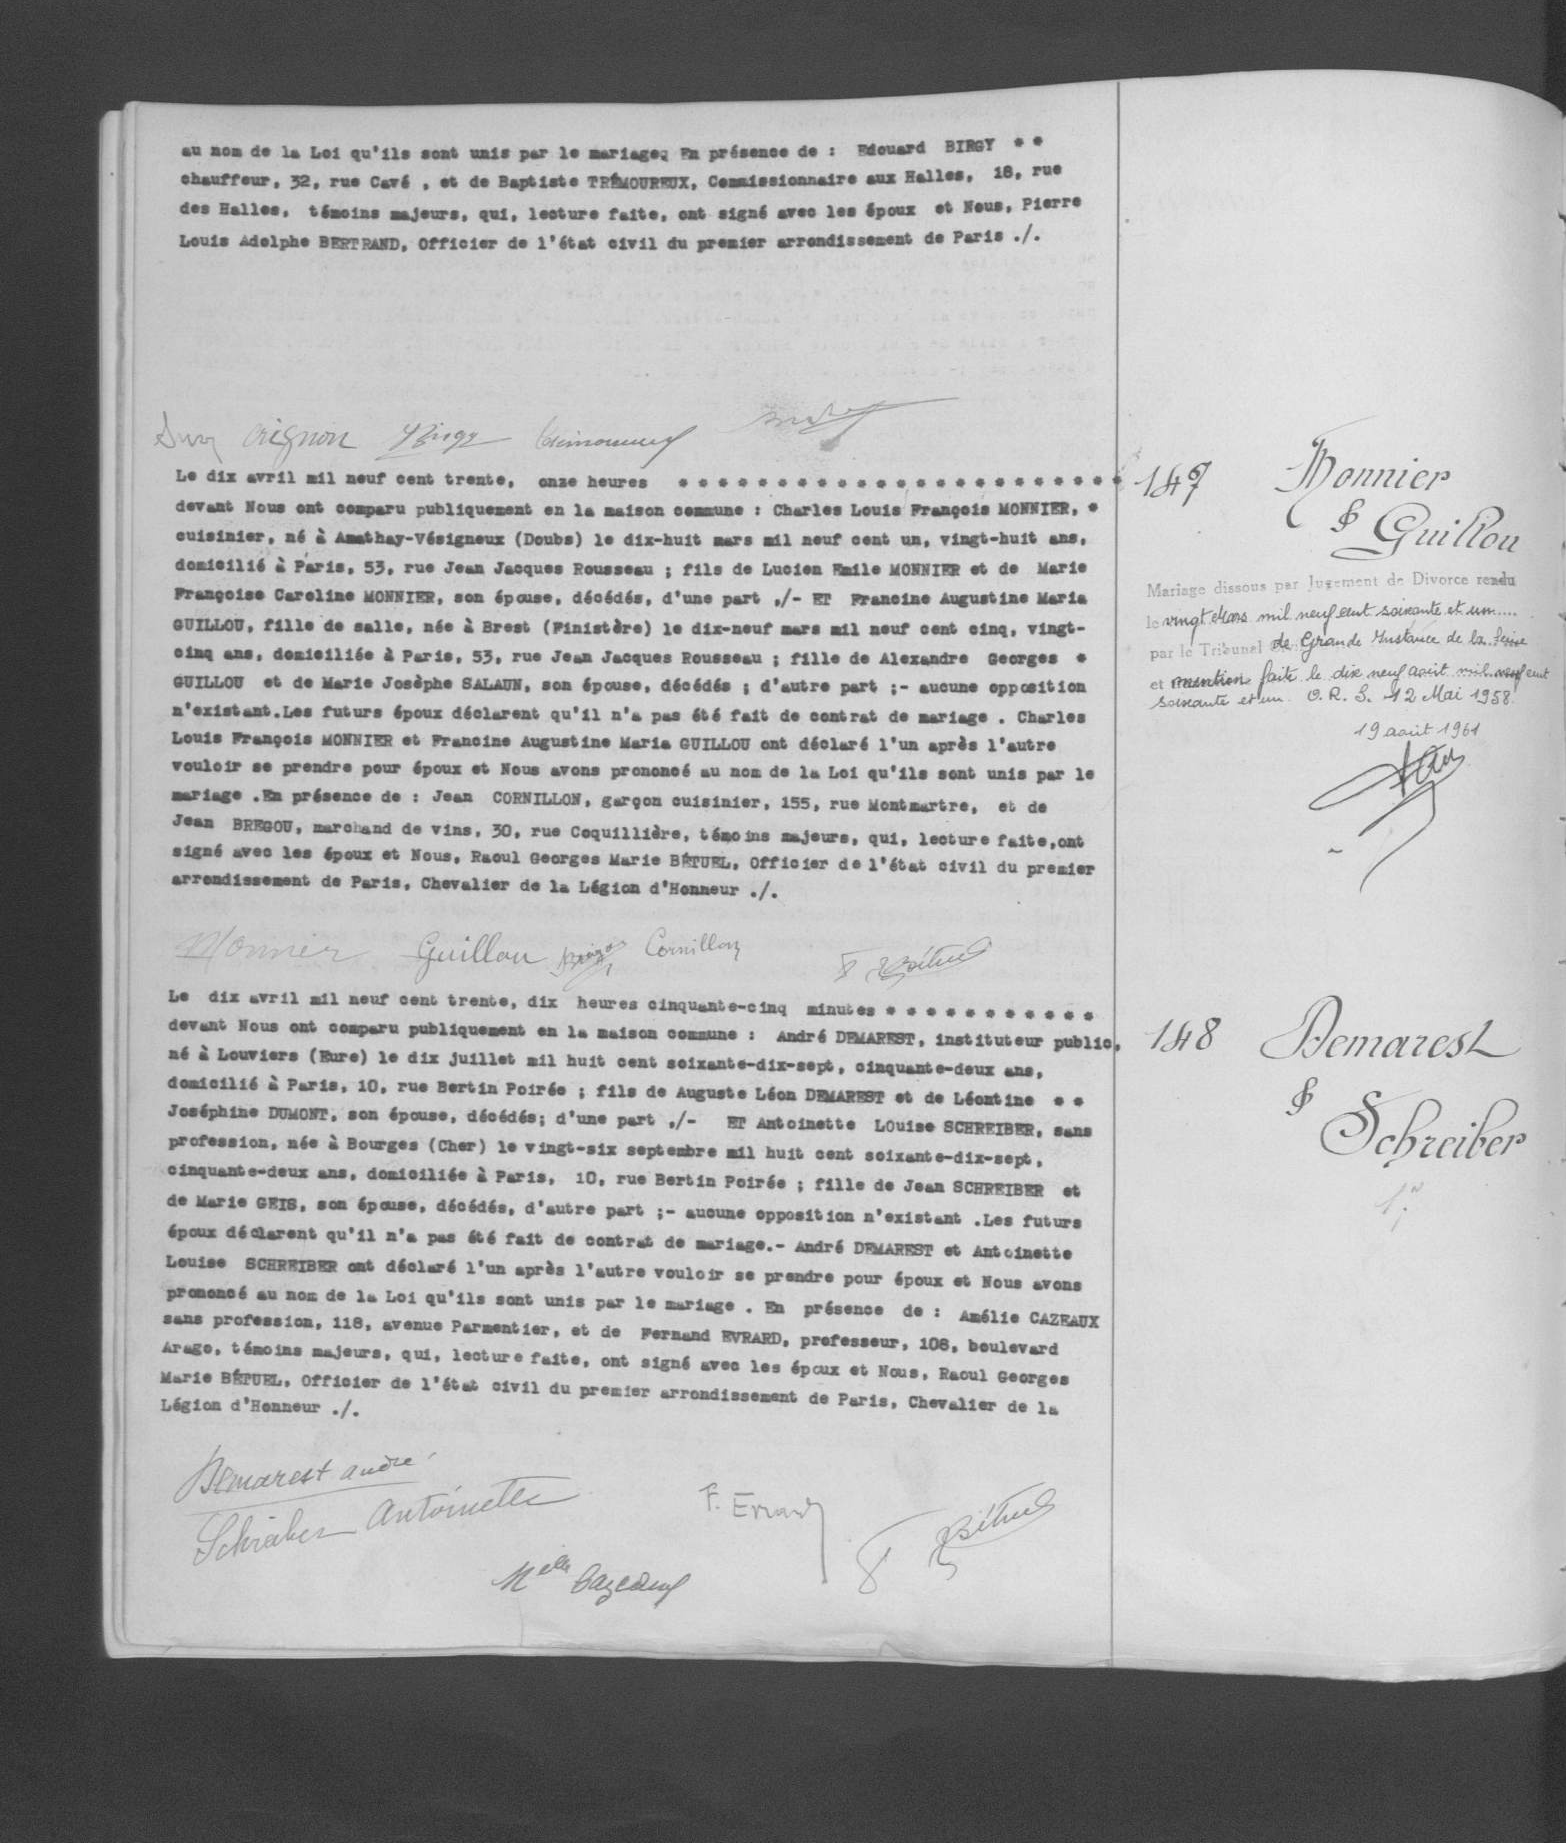au nom de la Loi qu'ils sont unis par le mariage. En présence de: Edouard BIRGY * *
chauffeur, 32, rue Cavé, et de Baptiste TREMOUREUX, Comissionnaire aux Halles, 18, rue
des Halles, témoins majeurs, qui, lecture faite, ont signé avec les époux et Nous, Pierre
Louis Adolphe BERTRAND, Officier de l'état civil du premier arrondissement de Paris ./.
Le dix avril mil neuf cent trente, onze heures ****
devant Nous ont comparu publiquement en la maison commune: Charles Louis François MONNIER, *
cuisinier, né à Amathay-Vésigneux (Doubs) le dix-huit mars mil neuf cent un, vingt-huit ans,
domicilié à Paris, 53, rue Jean Jacques Rousseau; fils de Lucien Emile MONNIER et de Marie
Françoise Caroline MONNIER, son épouse, décédés, d'une part ,/-ET Francine Augustine Maria
GUILLOU, fille de salle, née à Brest (Finistère) le dix-neuf mars mil neuf cent cinq, vingt-
cinq ans, domiciliée à Paris, 53, rue Jean Jacques Rousseau; fille de Alexandre Georges *
GUILLOU et de Marie Josèphe SALAUN, son épouse, décédés; d'autre part ;-aucune opposition
n'existant. Les futurs époux déclarent qu'il n'a pas été fait de contrat de mariage. Charles
Louis François MONNIER et Francine Augustine Maria GUILLOU ont déclaré l'un après l'autre
vouloir se prendre pour époux et Nous avons prononcé au nom de la Loi qu'il sont unis par le
mariage. En présence de: Jean CORNILLON, garçon cuisinier, 155, rue Montmartre, et de
Jean BREGOU, marchand de vins, 30, rue Coquillière, témoins majeurs, qui, lecture faite, ont
signé avec les époux et Nous, Raoul Georges Marie BETUEL, Officier de l'état civil du premier
arrondissement de Paris, Chevalier de la Légion d'Honneur ./.
Le dix avril mil neuf cent trente, dix heures cinquante-cinq minutes ****
devant Nous ont comparu publiquement en la maison commune: André DEMAREST, instituteur public,
né à Louviers (Eure) le dix juillet mil huit cent soixante-dix-sept, cinquante-deux ans,
domicilié à Paris, 10, rue Bertin Poirée; fils de Auguste Léon DEMAREST et de Léontine * *
Joséphine DUMONT, son épouse, décédéx; d'une part ,/-ET Antoinette Louise SCHREIBER, sans
profession, née à Bourges (Cher) le vingt-six septembre mil huit cent soixante-dix-sept,
cinquante-deux ans, domiciliée à Paris, 10, rue Bertin Poiée; fille de Jean SCHREIBER et
de Marie GEIS, son épouse, décédés, d'autre part ;-aucune opposition n'existant. Les futurs
époux déclarent qu'il n'pas été fait de contrat de mariage .-André DEMAREST et Antoinette
Louise SCHREIBER ont déclaré l'un après l'autre vouloir se prendre pour époux et Nous avons
prononcé au nom de la Loi qu'ils sont unis par le mariage. En présence de: Amélie CAZEAUX
sans profession, 118, avenue Parmentier, et de Fernand EVRARD, professeur, 108, boulevard
Arage, témoins majeurs, qui, lecture faite, ont signé avec les époux et Nous, Raoul Georges
Marie BETUEL, Officier de l'état civil du premier arrondissement de Paris, Chevalier de la
Légion d'Honneur ./.
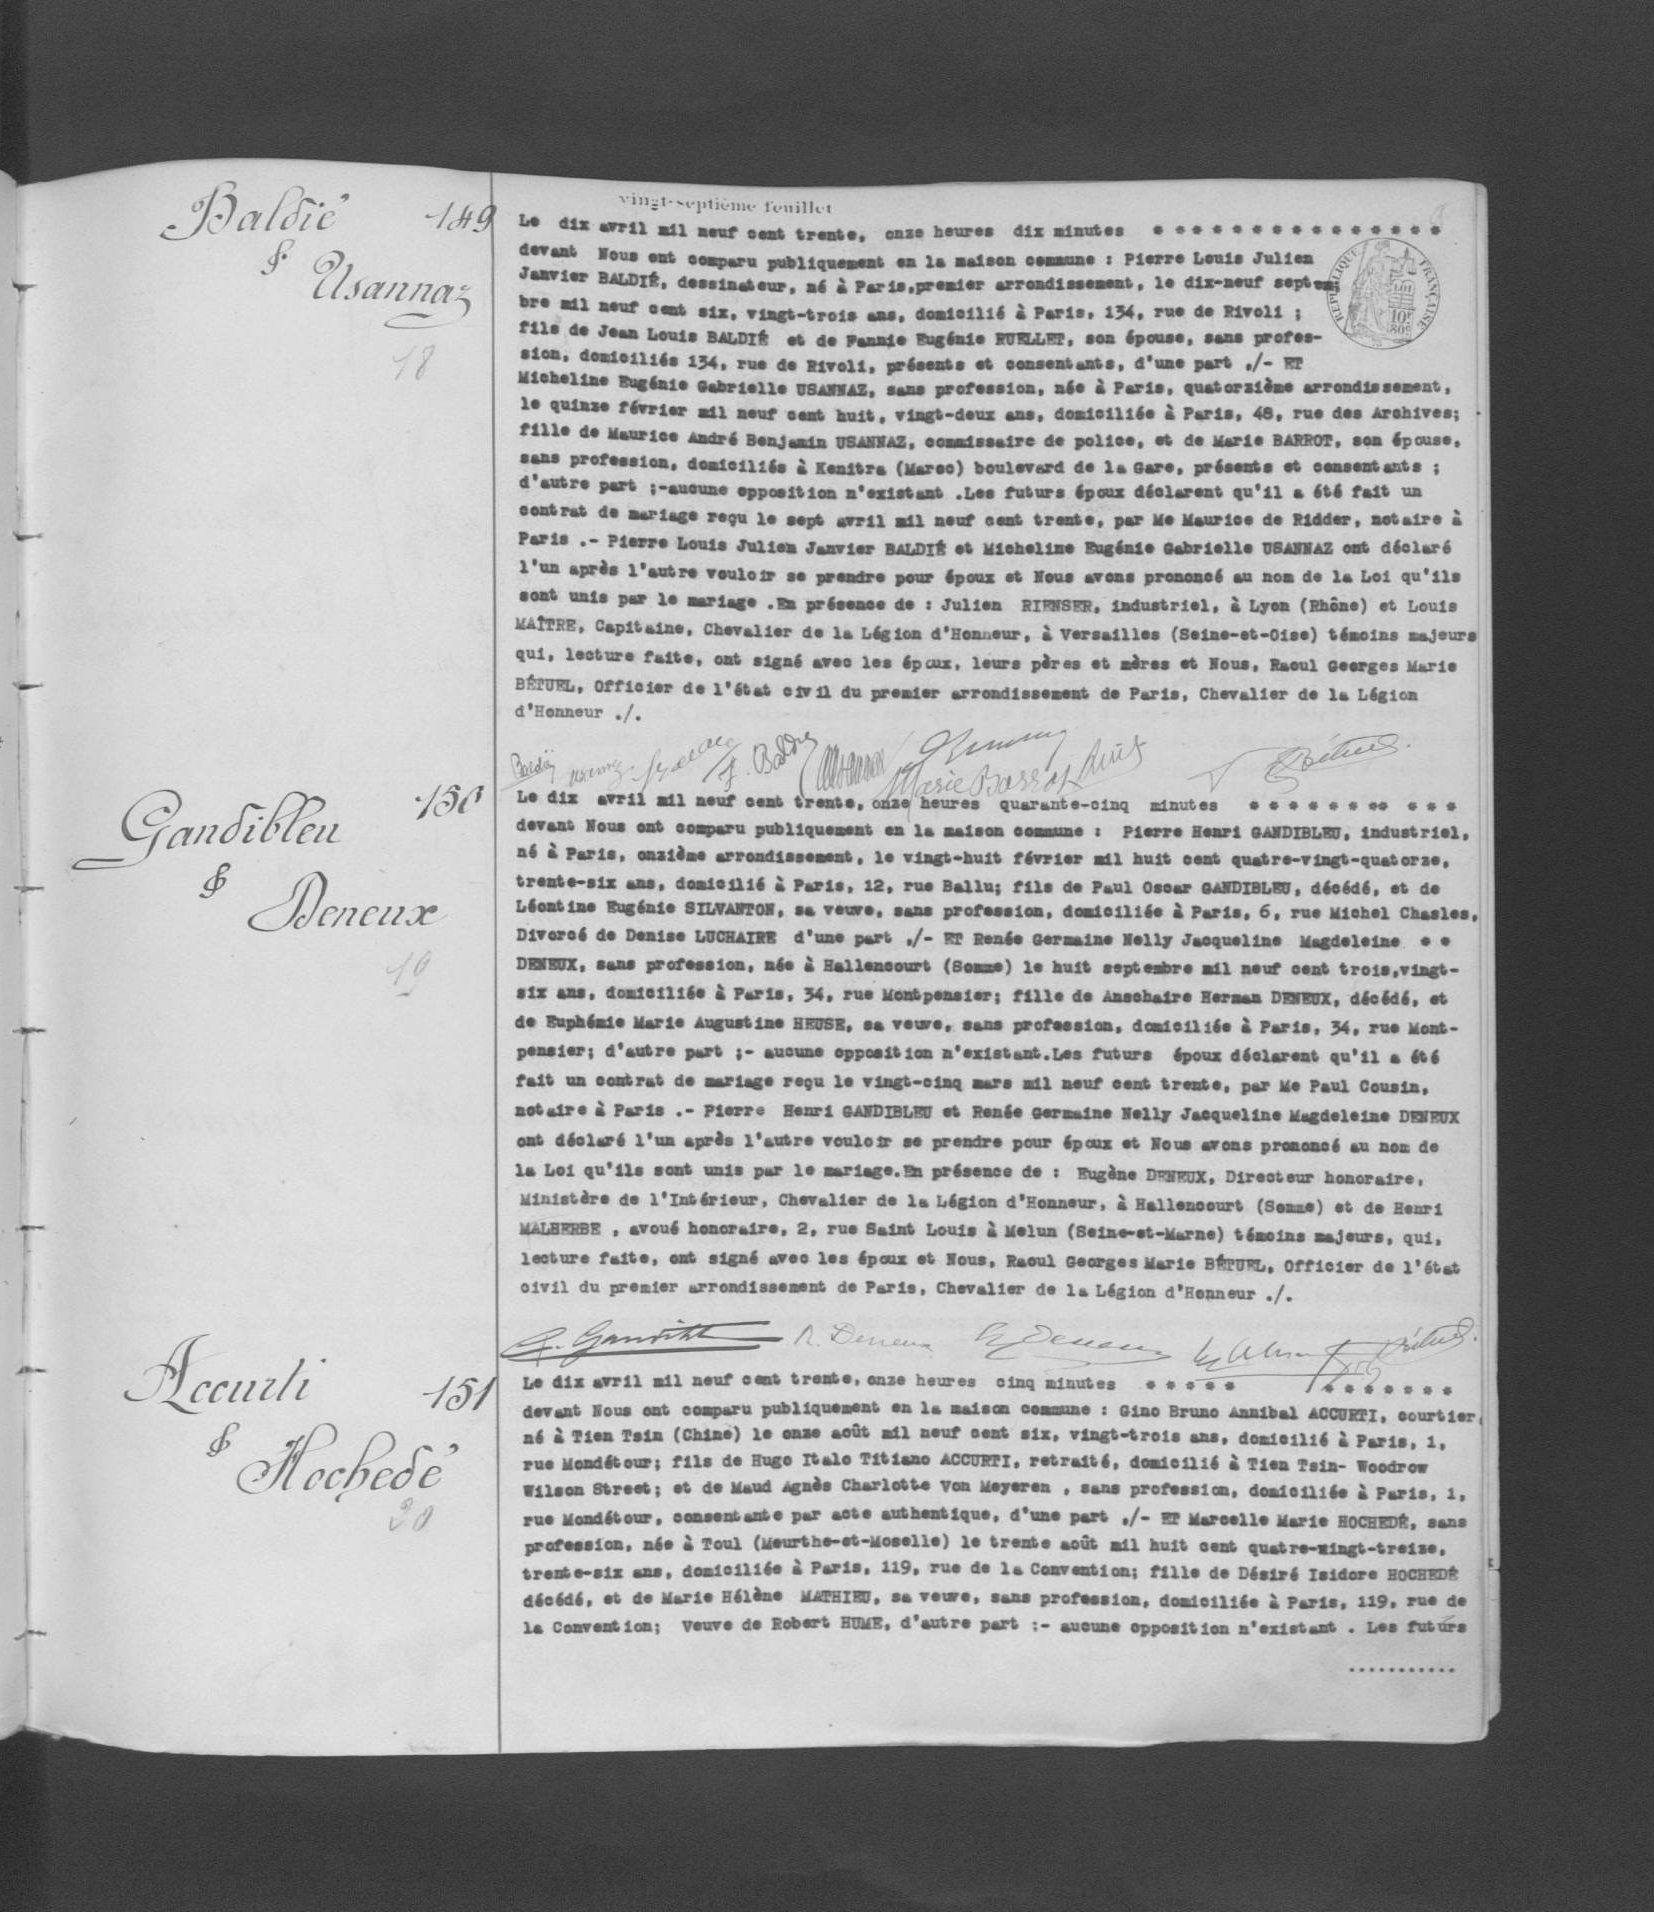Le dix avril mil neuf cent trente, onze heures dix minutes ****
devant Nous ont comparu publiquement en la maison commune: Pierre Louis Julien
Janvier BALDIE, dessinateur, né à Paris, premier arrondissement, le dix-neuf septem;
bre mil neuf cent six, vingt-trois ans, domicilié à Paris, 134, rue de Rivoli;
fils de Jean Louis BALDIE et de Fannie Eugénie RUELLET, son épouse, sans profes-
sion, domiciliés 134, rue de Rivoli, présents et consentants, d'une part ,/-ET
Micheline Eugénie Gabrielle USANNAZ, sans profession, née à Paris, quatorzième arrondissement,
le quinze février mil neuf cent huit, vingt-deux ans, domiciliée à Paris, 48, rue des Archives;
fille de Maurice André Benjamin USANNAZ, commissaire de police, et de Marie BARROT, son épouse,
sans profession, domiciliée à Kenitra (Maroc) boulevard de la Gare, présents et consentants;
d'autre part ;-aucune opposition n'existant. Les futurs époux déclarent qu'il a été fait un
contrat de mariage reçu le sept avril mil neuf cent trente, par Me Maurice de Ridder, notaire à
Paris .-Pierre Louis Julien Janvier BALDIE et Micheline Eugénie Gabrielle USANNAZ ont déclaré
l'un après l'autre vouloir se prendre pour époux et Nous avons prononcé au nom de la Loi qu'ils
sont unis par le mariage. En présence de: Julien RIENSER, industriel, à Lyon (Rhône) et Louis
MAITRE, Capitaine, Chevalier de la Légion d'Honneur, à Versailles (Seine-et-Oise) témoins majeurs
qui, lecture faite, ont signé avec les époux, leurs pères et mères et Nous, Raoul Georges Marie
BETUEL, Officier de l'état civil du premier arrondissement de Paris, Chevalier de la Légion
d'Honneur ./.
Le dix avril mil neuf cent trente, onze heures quarante-cinq minutes ****
devant Nous ont comparu publiquement en la maison commune: Pierre Henri GANDIBLEU, industriel,
né à Paris, onzième arrondissement le vingt-huit février mil huit cent quatre-vingt-quatorze,
trente-six ans, domicilié à Paris, 12, rue Ballu; fils de Paul Oscar GANDIBLEU, décédé, et de
Léontine Eugénie SILVANTON, sa veuve, sans profession, domiciliée à Paris, 6, rue Michel Chasles,
Divorcé de Denise LUCHAIRE d'une part ,/-ET Renée Germaine Nelly Jacqueline Magdeleine * *
DENEUX, sans profession, née à Hallencourt (Somme) le huit septembre mil neuf cent trois, vingt-
six ans, domiciliée à Paris, 34, rue Montpensier; fille de Anschaire herman DENEUX, décédé, et
Ephémie Marie Augustine HEUSE, sa veuve, sans profession, domiciliée à Paris, 34, rue Mont-
pensier; d'autre part ;-aucune opposition n'existant. Les futurs époux déclarent qu'il a été
fait un contrat de mariage reçu le vingt-cinq mars mil neuf cent trente, par Me Paul Cousin,
notaire à Paris .-Pierre Henri GANDIBLEU et Renée Germaine Nelly Jacqueline Magdeleine DENEUX
ont déclaré l'un après l'autre vouloir se prendre pour époux et Nous avons prononcé au nom de
la Loi qu'ils sont unis par le mariage. En présence de: Eugène DENEUX, Directeur honoraire,
Ministère de l'Intérieur, Chevalier de la Légion d'Honneur, à Hallencourt (Somme) et de Henri
MALHERBE, avoué honoraire, 2, rue Saint Louis à Melun (Seine-et-Marne) témoins majeurs, qui,
lecture faite, ont signé avec les époux et Nous, Raoul Georges Marie BETUEL, Officier de l'état
civil du premier arrondissement de Paris, Chevalier de la Légion d'Honneur ./.
Le dix avril mil neuf cent trente, onze heures cinq minutes ****
devant Nous ont comparu publiquement en la maison commune: Gino Bruno Annibal ACCURTI, courtier,
né à Tien Tsin (Chine) le onze août mil neuf cent six, vingt-trois ans, domicilié à Paris, 1,
rue Mondétour; fils de Hugo Italo Titiano ACCURTI, retraité, domicilié à Tien Tsin-Woodrow
Wilson Street; et de Maud Agnès Charlotte Von Meyeren, sans profession, domiciliée à Paris, 1,
rue Mondétour, consentante par acte authentique, d'une part ,/-ET Marcelle Marie HOCHEDE, sans
profession, née à Toul (Meurthe-et Moselle) le trente août mil huit cent quatre-vingt-treize,
trente-six ans, domicilié à Paris, 119, rue de la Convention; fille de Désiré Isidore HOCHEDE
décédé, et de Marie Hélène MATHIEU, sa veuve, sans profession, domiciliée à Paris, 119, rue de
la Convention; veuve de Robert HUME, d'autre part ;-aucune opposition n'existant. Les futurs